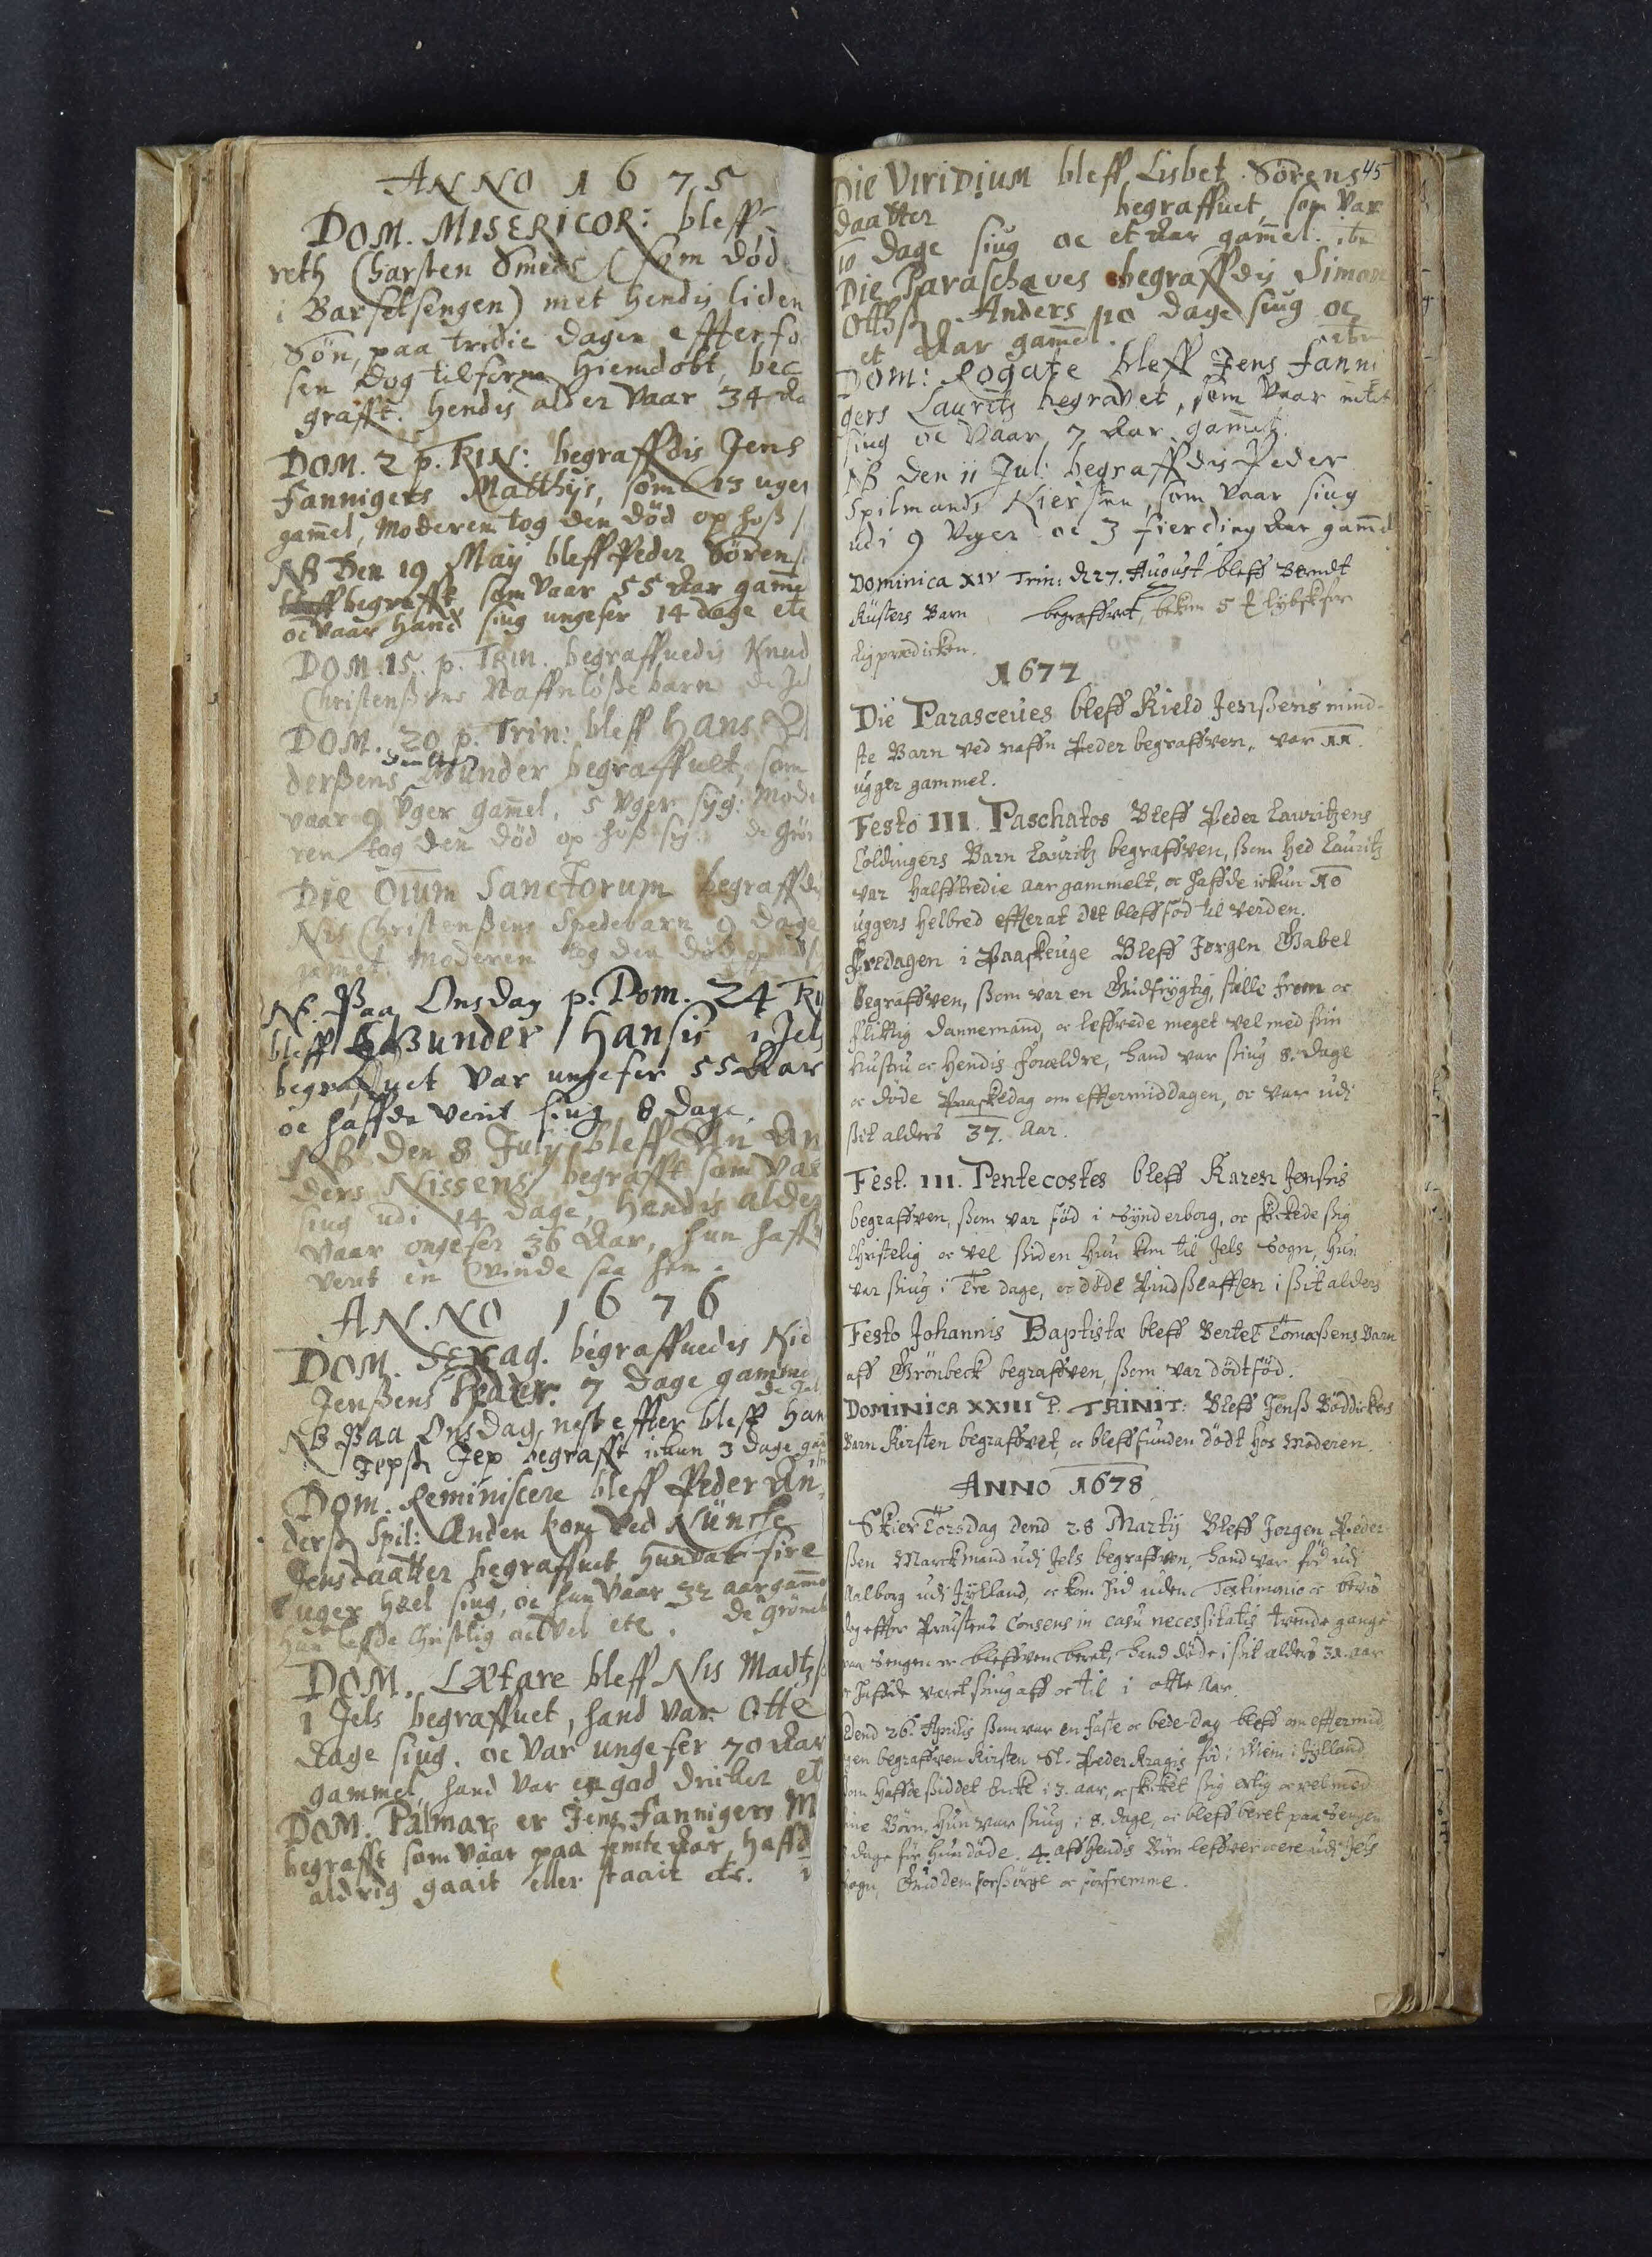ANNO 1675
DOM. MISERICOR: bleff
reth Charsten Smeds (som døde
i Barselsengen) met hendis liden 
Søn, paa tridie Dagen effter fo
sen dog tilforne Hiemdøbt, be¬
grafft, hendis alder vaar 34 Aa 
DOM. 2 p. TRIN: begraffdis Jens
Fannigers Matthijs, som 13 uger
gammel, Moderen tog den død op hoss s 
NB Den 19 Maij bleff Peder Søren
bleff begrafft som vaar 55 Aar gamme 
oc vaar Hand siug ungefer 14 dage etc
DOM. 15 p. Trin. begraffuedis Knud
Christenssøns naffnløsse barn de Jel 
DOM. 20 p. Trin. bleff Hans A 
datter
dersens Gunder begraffuet, som
vaar 9 Vger gammel, 5 Vger syg: Mode
ren tog den død op hoss sig. De Grø 
Die Oium## Sanctorum begraffdis
Nis Christenssens Spedebarn 9 dage
gammel. Moderen tog den død op
NB Paa Onsdag p. Dom. 24 Tr 
bleff Gunder Hansis i Jels
begraffuet var ungefer 55 Aar
oc haffde verit siug 8 Dage.
NB Den 8 Julij bleff An An
ders Nissens begrafft som var
siug udi 14 Dage, hendis alder
vaar ungefer 36 Aar, hun
veret en Qvinde saa hen
ANNO 1676
DOM. Sexag. begraffuedes Kie 
Jenssens Peder. 7 Dage gamme 
de Jels
NB Paa Onsdag, nest effter bleff Han 
Jepss Jep begrafft ickun 3 dage
Ibm
Dom. Reminiscere bleff Peder An¬
derss Spil: Anden Kone Ved Nunche
Jensdaatter begraffuet, Hun var fire
uger Heel## siug, oc hun vaar 32 aar gamme 
Hun leffde Christlig oc Vel etc. de Grøneb 
DOM. Latare bleff Nis Madtzso 
i Jels begraffuet, hand var otte
dage siug, oc var ungefer 70 Aar
gammel, hand var en god dricker et 
DOM. Palmar er Jens Fannigers M 
begrafft som vaar paa femte Aar, haffd 
aldrig gaait eller staait etc.
45
Die VIRIDIUM bleff Lisbet Sørens
Daatter begraffuet som var
10 Dage siug oc et Aar gammel. ibm
Die Paraschaves begraffdis Simon 
Otthss Anders 10 dage siug oc
et Aar gammel.
ibm.
Dom: Rogate bleff Jens Fanni
gers Laurits begravet, som vaar intet
siug oc vaar 7 Aar gammel.
NB Den 11 Jul: begraffdis Peder
Spilmands Kiersten, som vaar siug
udi 9 Vger oc 3 fierding Aar gammel
Dominica XIV Trin: D 27. August bleff Berndt
Kusters Barn begraffvet, bekom 5 ## lybsk for
ligprædicken.
1677
Die Parasceues bleff Kield Jenssens mind¬
ste Barn ved naffn Peder Begraffven, var 11
ugger gammel.
Festo III Paschatos Bleff Peder Lauritzens
Coldingers Barn Lauritz begraffven, som hed Lauritz
var halfftredie Aar gammelt, oc haffde ickun 10
uggers helbred effter at det bleff fød til verden.
Fredagen i Paaskeuge Bleff Jørgen Gabel
Begraffven, ssom var en Gudfrygtig, stille from oc
flittig Dannemand, oc lefvede meget vel med sin
hustru oc hendis forældre, hand var siug 8 dage
oc døde Paaskedag om efftermiddagen, oc var udi
ssit alders 37. Aar.
Fest. III Pentecostes bleff Karen Jensis
begraffven, ssom var fød i Synderborg, oc skickede sig
Christelig oc vel ssiden Hun kom til Jels sogn, Hun
var siug i Tre dage, oc døde Pindsseaften i ssit alders
Festo Johannis Baptista bleff Bertel Tomassens Barn
aff Grønbeck begraffven, ssom var dødtfød.
DOMINICA XXIII P. TRINITY: Bleff Jens Bøddickers
Barn Kirsten Begraffvet, oc bleff funden dødt hos Moderen.
ANNO 1678
Skier Torsdag dend 28 Martij Bleff Jørgen Peder
ssen Marckmand udi Jels begraffven, hand var fød udi
Aalborg udi Jylland, oc kom hid uden Testimonio oc bevis
dog effter Prustens Consens in casu necessitatis tvende gange
paa sengen er bleffwen beret, hand døde i sit alders 31 aar
oc hafde været siug aff oc til i otte Aar.
Dend 26. Aprilis som var en faste oc bededag bleff om efftermid¬
gen begraffven Kirsten Sl. Peder Kragis fød i Wein i Jylland
ssom haffde ssiddet Encke i 3. aar, oc skicket ssig erlig oc vel mod
ssine Børn, hun var siug i 8. Dage, oc bleff beret paa Sengen
8. dage før Hun døde. 4. aff hendis Børn leffver oc ere udi Jels
Sogn. Gud dem forsørge oc forfremme